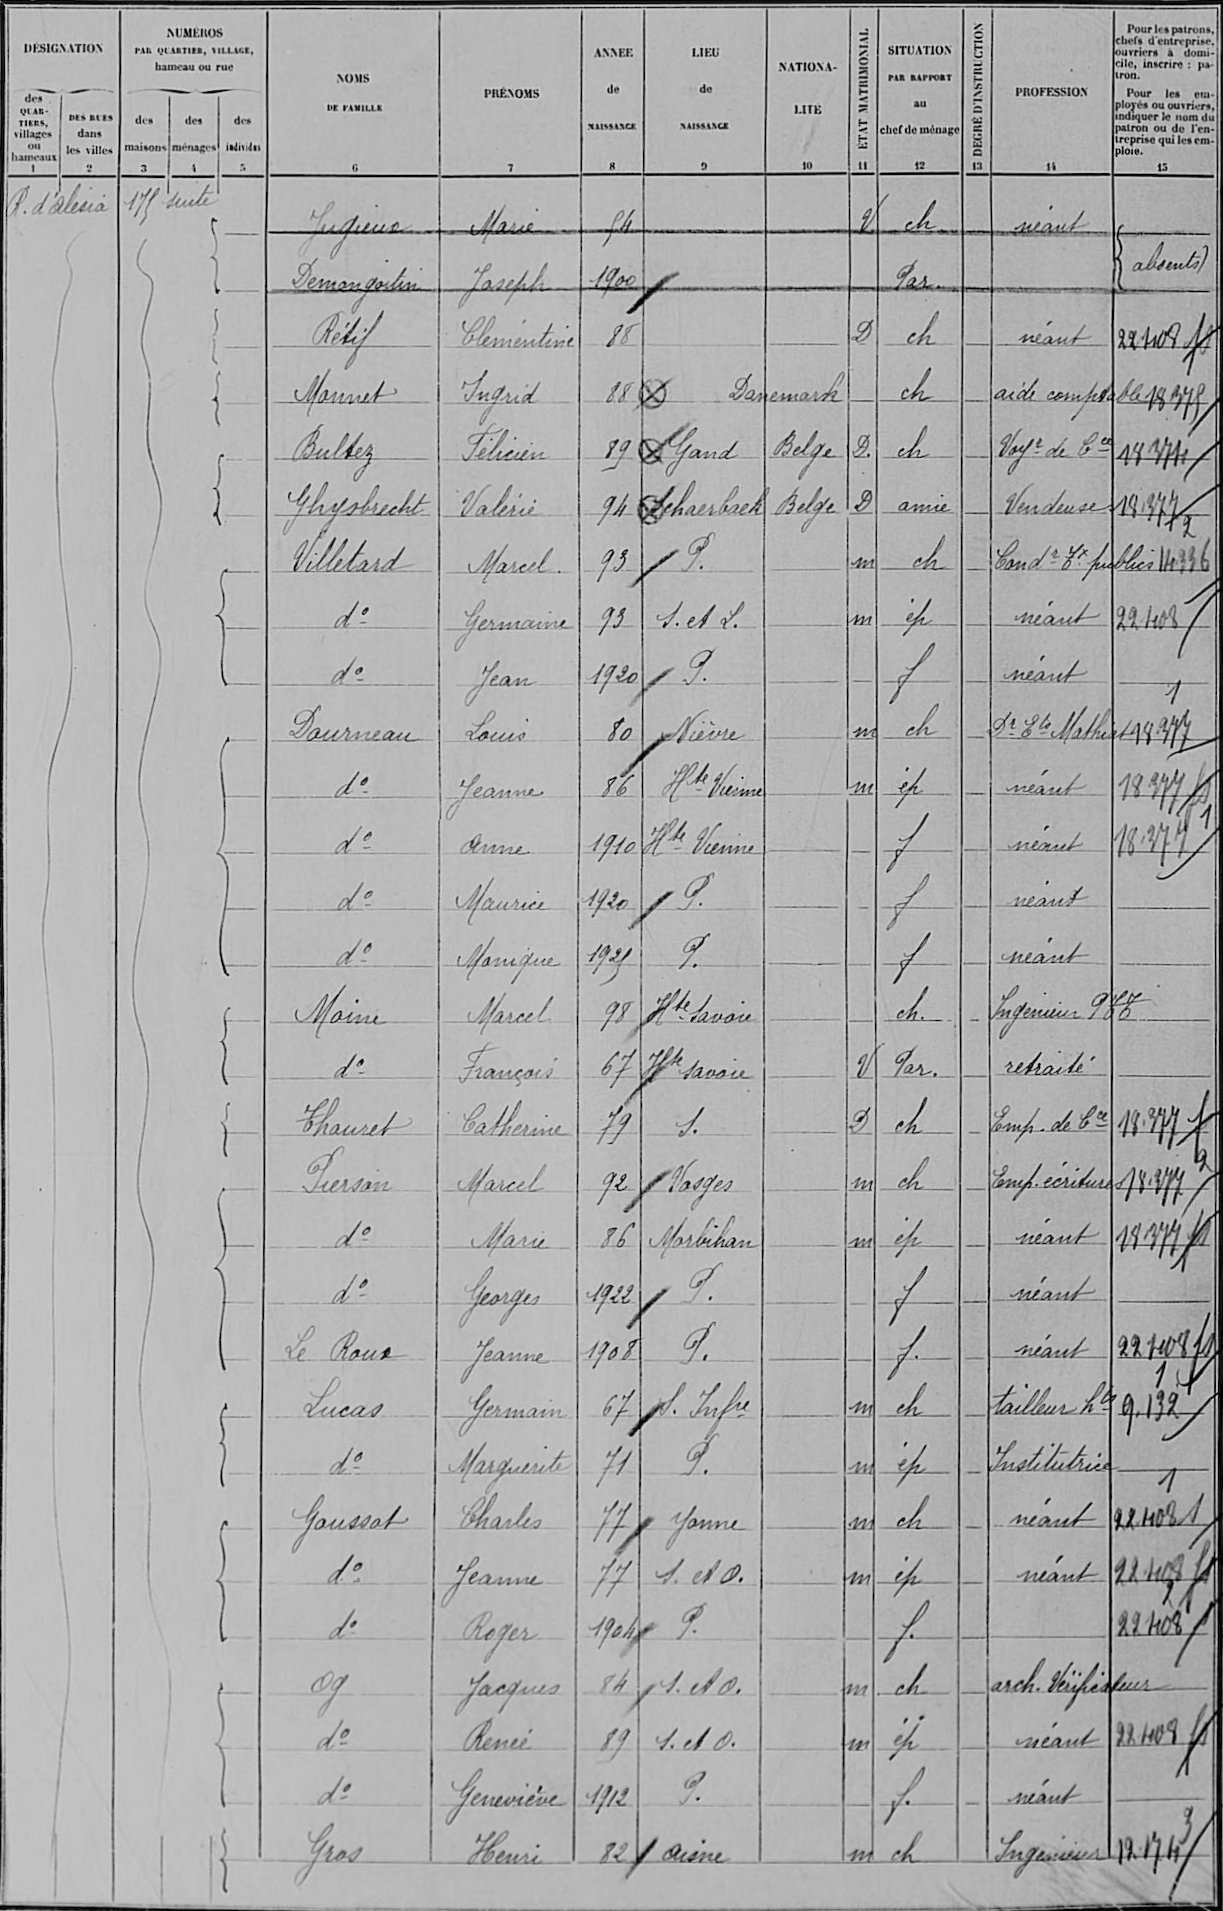Jugieux/Marie/54/¤/¤/V /ch/¤/néant/¤
Demongoitin/Joseph/1900/¤/¤/¤/Par/¤/¤/absents)
Réhif/Clementine/88/¤/¤/D/ch/¤/néant/22408
Monnet/Ingrid/88/Danemark/¤/¤/ch/¤/aide comptable/18375
Bulhez/Félicien/89/Gand/Belge/D /ch/¤/Voyr de Cce/18374
Ghysbrecht/Valérie/94/Schaerbaek/Belge/D/amie/¤/Vendeuse/18377 2
Villetard/Marcel /93/P /¤/m/ch/¤/Condr tx publics/14336
d°/Germaine/93/S et L /¤/m/ép/¤/néant/22408
d°/Jean/1920/P /¤/¤/f/¤/néant/¤
Dourneau/Louis/80/Nièvre/¤/m/ch/¤/Dr Ets Mathiat/18 377
d°/Jeanne/86/Hte Vienne/¤/m/ép/¤/néant/18377 1
d°/anne/1910/Hte Vienne/¤/¤/f/¤/néant/18 377 1
d°/Maurice/1920/P /¤/¤/f/¤/néant/¤
d°/Monique/1921/P /¤/¤/f/¤/néant/¤
Moine/Marcel/98/Hte Savoie/¤/¤/ch /¤/Ingénieur PTT/¤
d°/François/67/Hte Savoie/¤/V/Par /¤/retraité/¤
Thauret/Catherine/79/S /¤/D/ch/¤/Emp de Cce/18 377
Pierson/Marcel/92/Vosges/¤/m/ch/¤/Emp écritures/18 377 2
d°/Marie/86/Morbihan/¤/m/ép/¤/néant/18377
d°/Georges/1922/P /¤/¤/f/¤/néant/¤
Le Roux/Jeanne/1908/P /¤/¤/f /¤/néant/22408
Lucas/Germain/67/S Infre/¤/m/ch/¤/tailleur hts/9 132
d°/Marguerite/71/P /¤/m/ép/¤/Institutrice/¤
Goussot/Charles/77/Yonne/¤/m/ch/¤/néant/22 408 1
d°/Jeanne/77/S et O /¤/m/ép/¤/néant/22 408 2
d°/Roger/1904/P /¤/¤/f /¤/¤/22408
Og/Jacques/84/S et O /¤/m/ch/¤/arch Vérificateur/¤
d°/Renée/89/S et O /¤/m/ép/¤/néant/22408
d°/Geneviève/1912/P /¤/¤/f /¤/néant/¤
Gros/Henri/82/aisne/¤/m/ch/¤/Ingénieur/12174 3
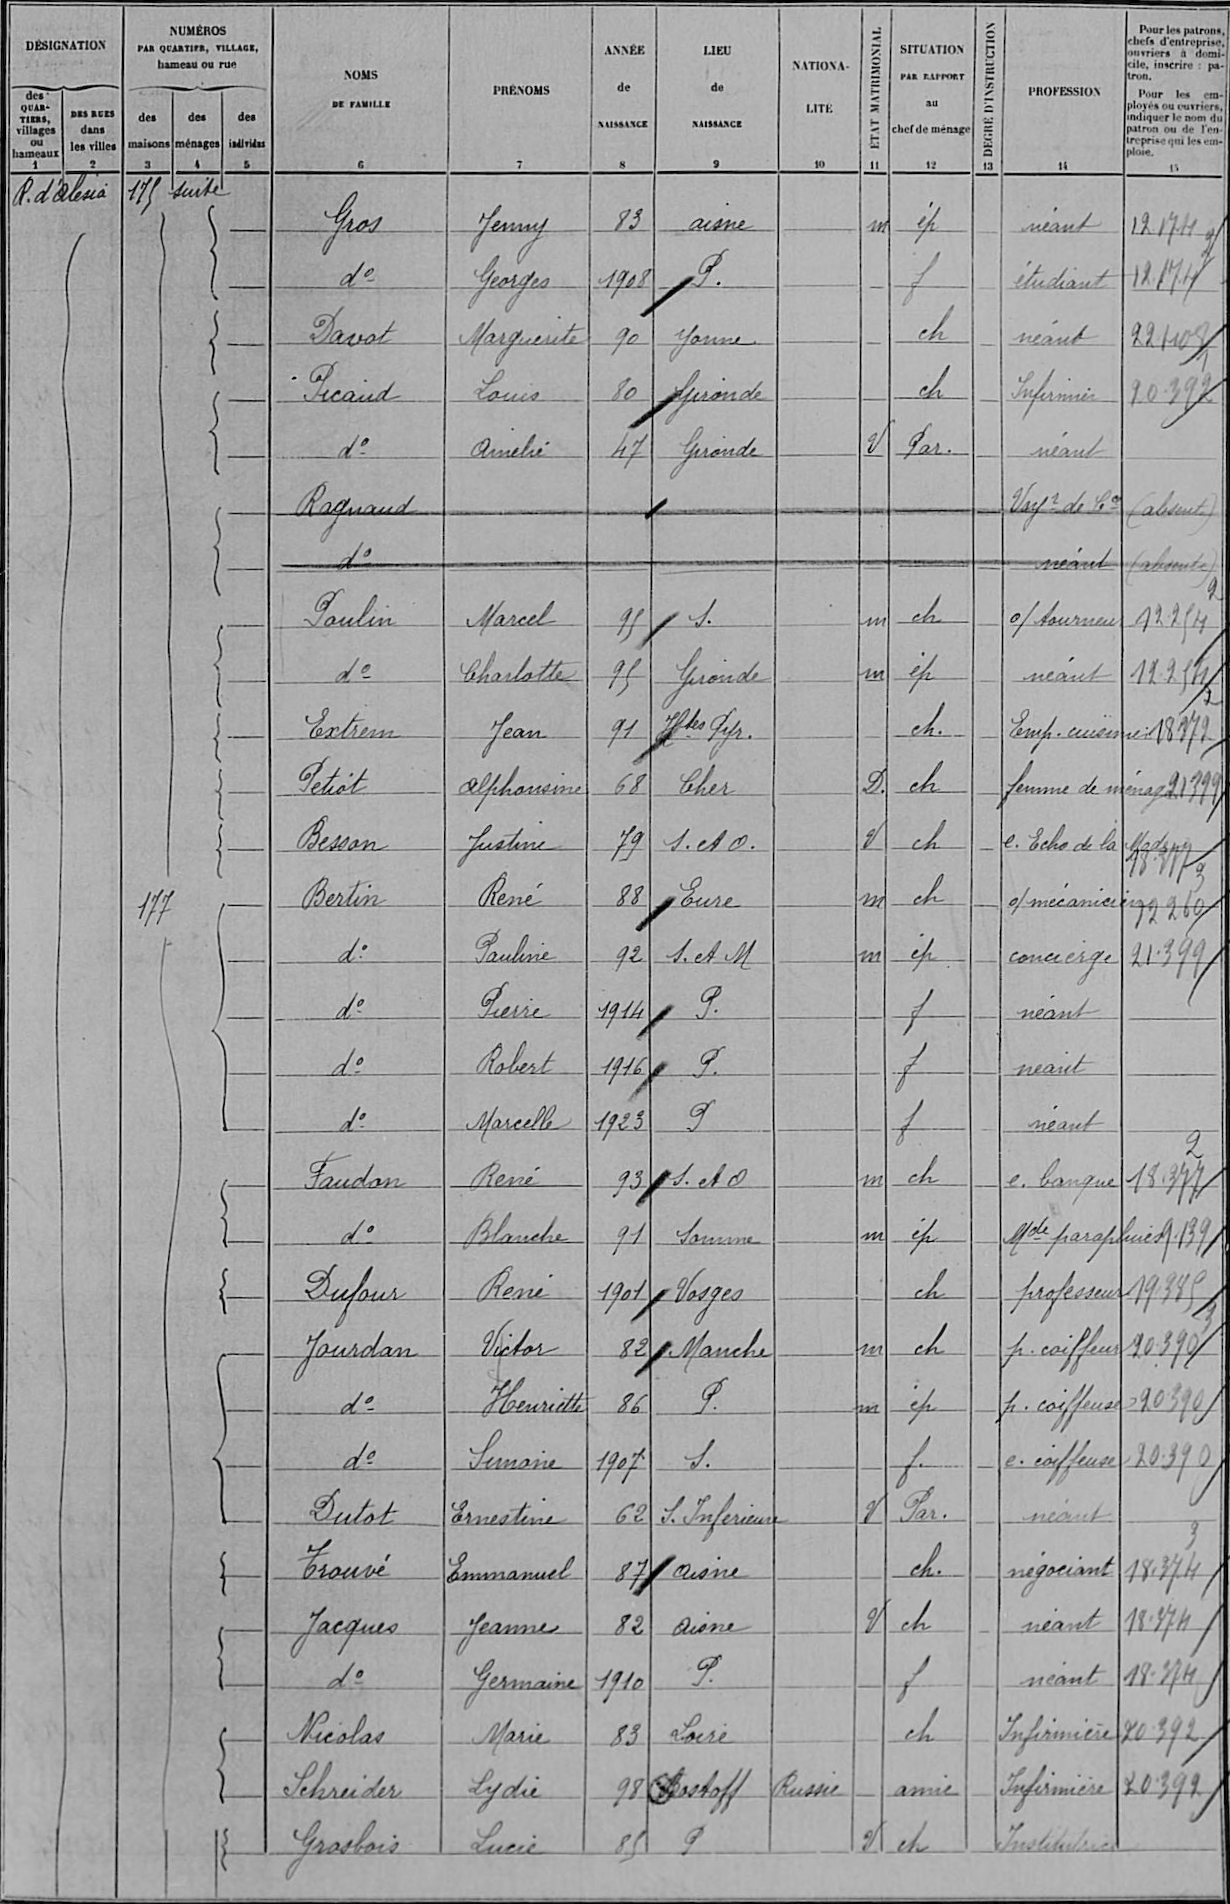Gros/Jenny/83/aisne/¤/m/ép/¤/néant/12174
d°/Georges/1908/P /¤/¤/f/¤/étudiant/12174
Davot/Marguerite/90/Yonne/¤/¤/ch/¤/néant/22408
Picaud/Louis/80/Gironde/¤/¤/ch/¤/infirmier/20392
d°/amélie/47/Gironde/¤/V/Par /¤/néant/¤
Ragnaud/¤/¤/¤/¤/¤/¤/¤/Voyr de Cce/(absente)
d°/¤/¤/¤/¤/¤/¤/¤/néant/(absente)
Poulin/Marcel/95/S /¤/m/ch/¤/o tourneur/12254
d°/Charlotte/95/Gironde/¤/m/ép/¤/néant/12254
Extrem/Jean/91/Htes Pyr /¤/¤/ch /¤/Emp cuisine/18372
Petiot/Alphonsine/68/Cher/¤/D /ch/¤/femme de ménage/21399
Besson/Justine/79/s et O /¤/V/ch/¤/e echo de la mode/18377
Bertin/René/88/Eure/¤/m/ch/¤/o mécanicien/22260
d°/Pauline/92/S et M/¤/m/ép/¤/concierge/21399
d°/Pierre/1914/P /¤/¤/f/¤/néant/¤
d°/Robert/1916/P /¤/¤/f/¤/néant/¤
d°/Marcelle/1923/P/¤/¤/f/¤/néant/¤
Faudon/René/93/S et O/¤/m/ch/¤/e banque/18377
d°/Blanche/91/Somme/¤/m/ép/¤/Mde parapluie/9139
Dufour/René/1901/Vosges/¤/¤/ch/¤/professeur/19389
Jourdan/Victor/82/Manche/¤/m/ch/¤/pr coiffeur/10390
d°/Henriette/86/P /¤/m/ép/¤/pr coiffeuse/20390
d°/Simone/1907/S /¤/¤/f /¤/e coiffeuse/20390
Dutot/Ernestine/62/S Inferieure/¤/V/Par /¤/néant/¤
Trouvé/Emmanuel/87/aisne/¤/¤/ch /¤/négociant/18374
Jacques/Jeanne/82/aisne/¤/V/ch/¤/néant/18374
d°/Germaine/1910/P /¤/¤/f/¤/néant/18374
Nicolas/Marie/83/Loire/¤/¤/ch/¤/Infirmière/20392
Schreider/Lydie/98/Kostoff/Russie/¤/amie/¤/Infirmière/20392
Grosbois/Lucie/85/P/¤/V/ch/¤/Institutrice/¤